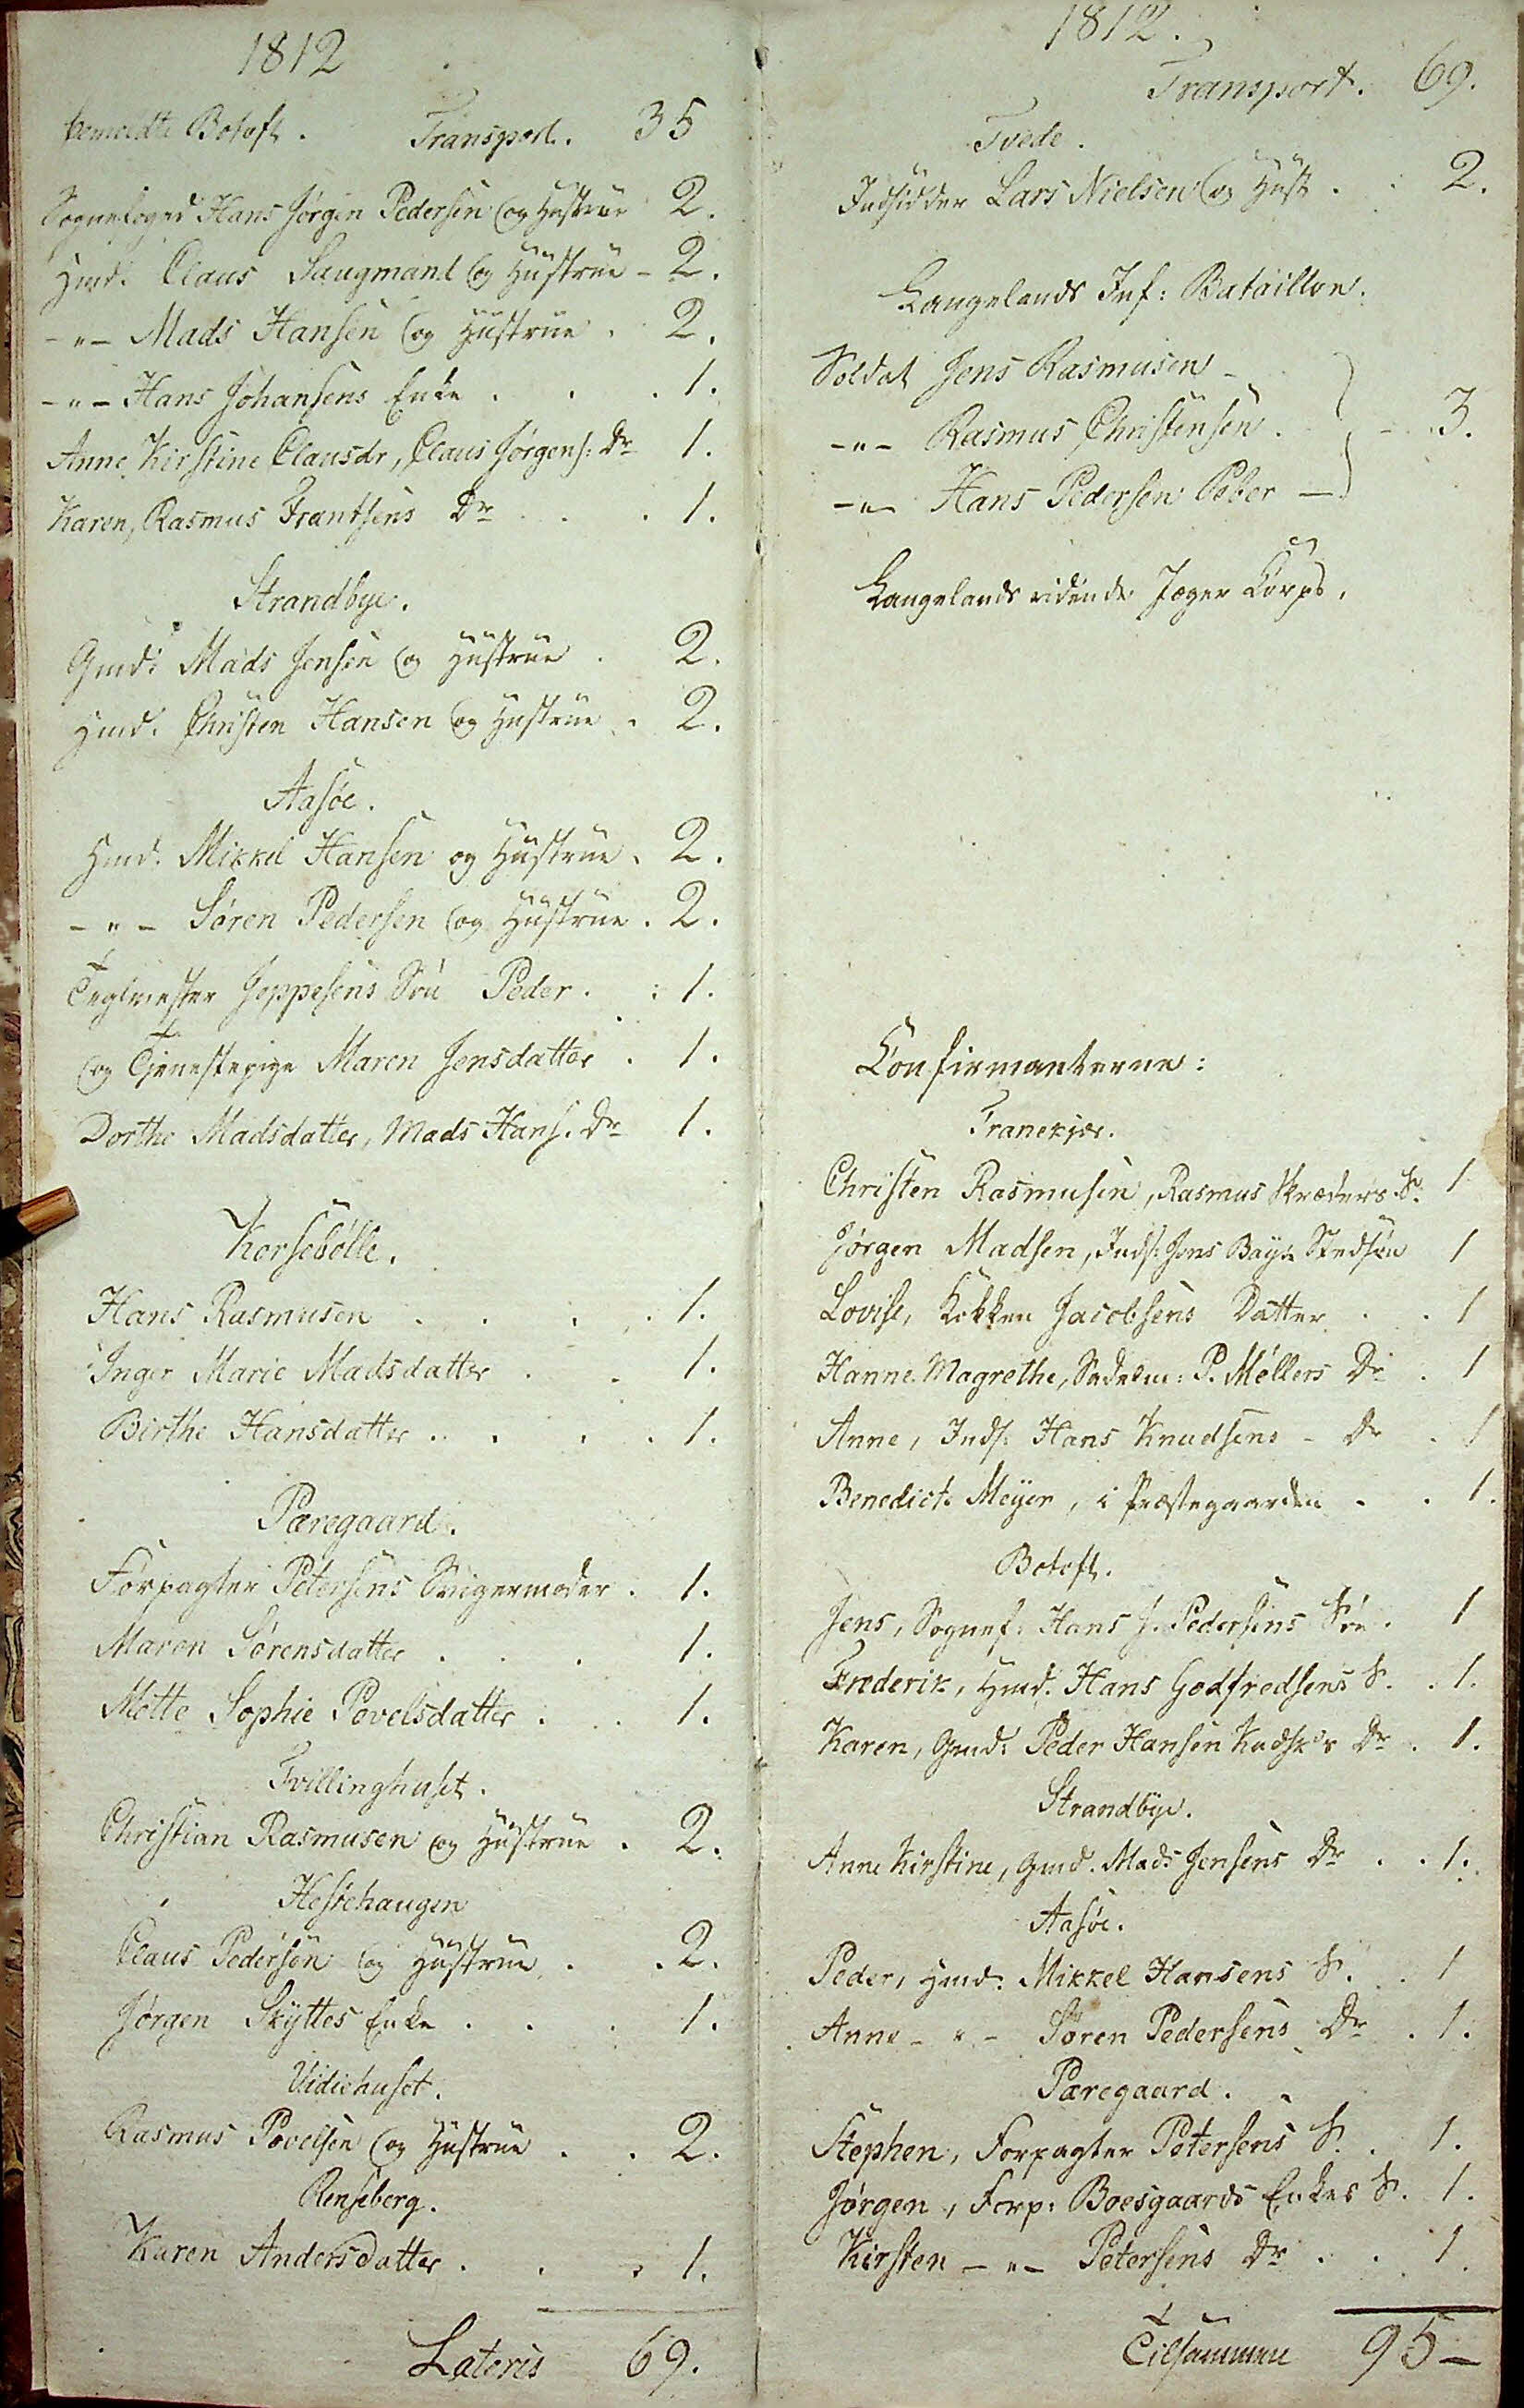1812
bemeldte Botoft. Transport. 35
Sognefoged Hans Jørgen Pedersen og Hustrue 2.
Hmd: Claus Saugmand og Hustrue - 2.
-"- Mads Hansen og Hustrue . 2.
-"- Hans Johansens Enke  . . . 1.
Anne Kirstine Clausdr, Claus Jørgens: Dr 1.
Karen, Rasmus Frantzens Dr . . . 1.
Strandbye.
Gmd. Mads Jensen og Hustrue . 2.
Hmd. Christen Hansen og Hustrue . 2.
Aasøe
Hmd. Mikkel Hansen og Hustrue . 2.
-"- Søren Pedersen og Hustrue . 2.
Teglmester Jeppesens Søn Peder . : 1.
og Tjenestepige Maren Jensdatter . 1.
Dorthe Madsdatter, Mads Hans. Dr 1.
Korsebølle.
Hans Rasmusen . . . . 1.
Inger Marie Madsdatter . . 1.
Birthe Hansdatter . . . . 1.
Pæregaard.
Forpagter Petersens Svigermoder . 1.
Maren Sørensdatter . . . 1.
Mette Sophie Povelsdatter . . . 1.
Tvillinghuset
Christian Rasmusen og Hustrue . 2.
Hestehaugen
Claus Pedersens og Hustrue . . 2.
Jørgen Skyttes Enke . . . 1.
Vidiehuset.
Rasmus Povelsen og Hustrue . . 2.
Renseberg.
Karen Andersdatter . . . 1.
Lateris 69.
1812
Transport. 69.
Tvede
Indsidder Lars Nielsens og Hust . . 2.
Langelands Inf: Bataillon.
Soldat Jens Rasmusen
-"- Rasmus Christensen
-"- Hans Pedersen ##ber
3.
Langelands ridende Jæger Corps.
Konfirmanterne:
Tranekjær
Christen Rasmusen, Rasmus Skræders S . 1
Jørgen Madsen, Jnds: Jens Bays Stedsøn 1
Lovise, Kokken Jacobsens Datter . . 1
Hanne Magrethe, Sadelm: P. Møllers Dr 1
Anne, Inds: Hans Knudsens - Dr . 1
Bendicte Meyer, i Præstegaarden . . 1
Botoft.
Jens, Sognef: Hans J. Pedersens Søn . 1
Frederik, Hmd. Hans Godfredsens S . . 1.
Karen, Gmd: Peder Hansen Kudsk's Dr . 1.
Stranbye.
Anne Kirstine, Gmd. Mads Jensens Dr . . 1.
Aasøe.
Peder, Hmd Mikkel Hansens S . . . 1
Anne -"- Jøren Pedersens Dr . 1.
Pæregaard.
Stephen, Forpagter Petersens S . . 1.
Jørgen, Forp: Boesgaards Enkes S .1.
Kirsten -"- Petersens Dr . . 1.
Tilsammen 95 -
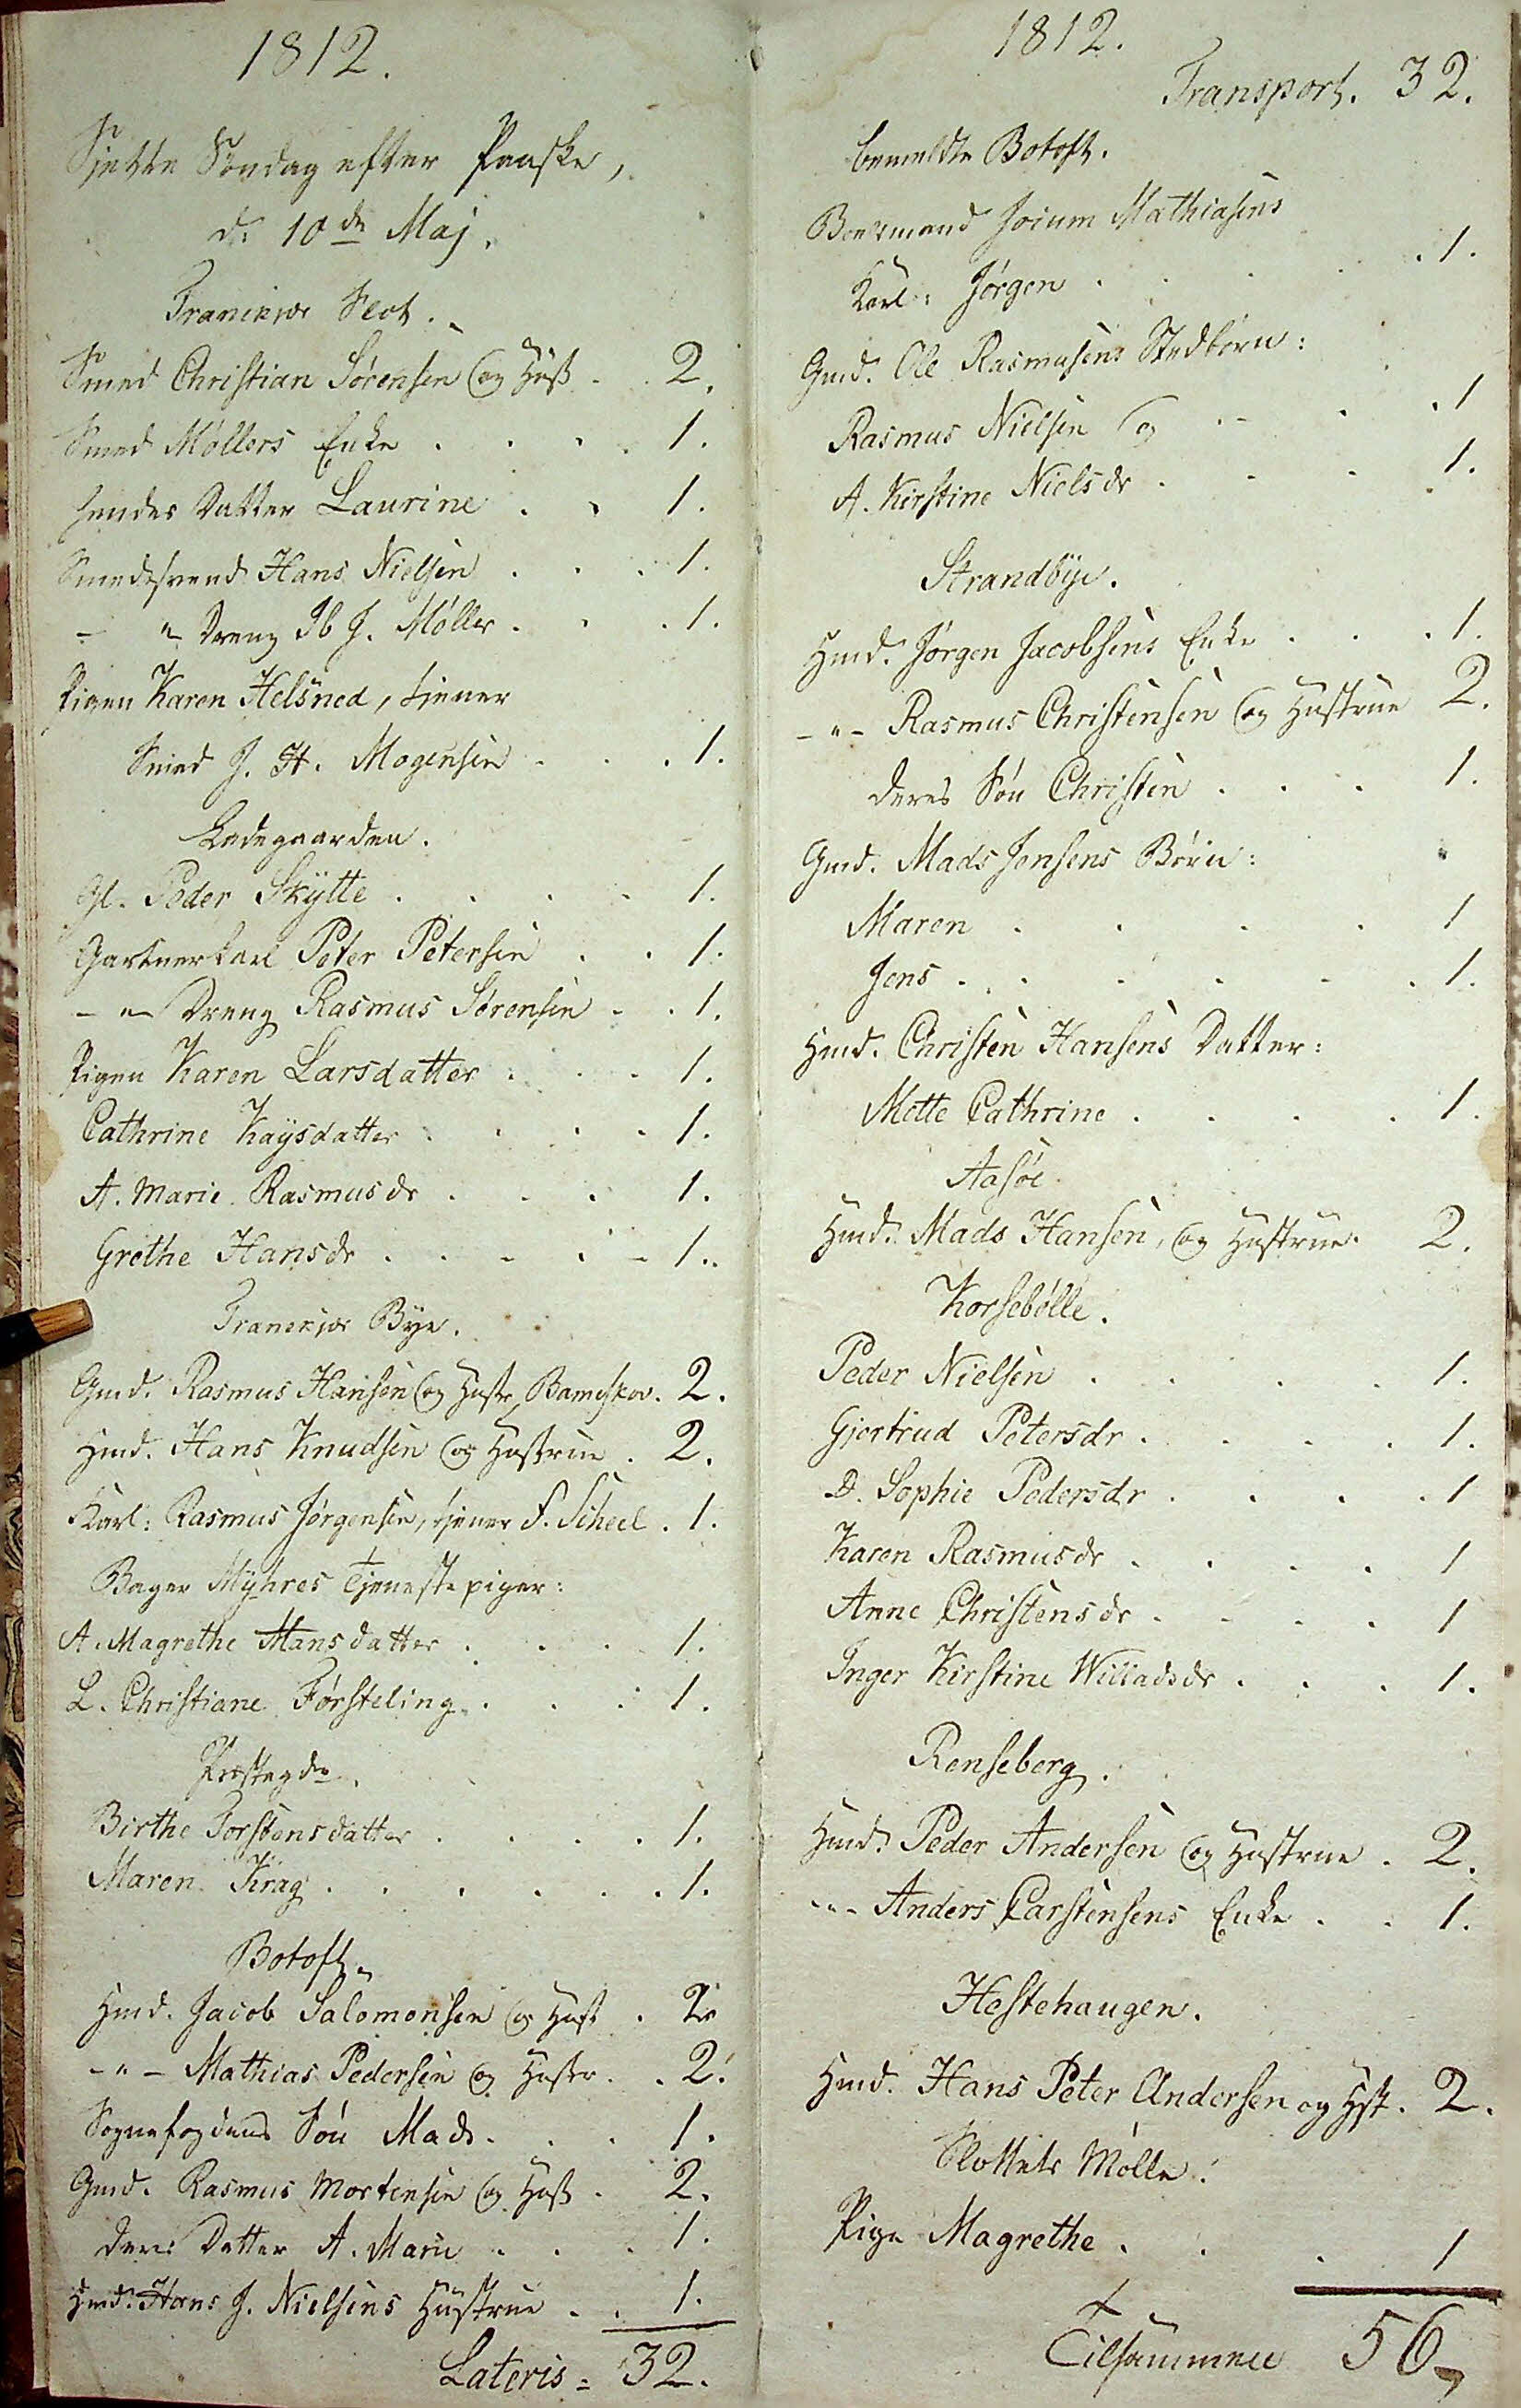1812.
Sjette Søndag efter Paaske,
d. 10de Maj
Tranekjær Slot.
Smed Christian Sørensen og Hust . . 2.
Smed Møllers Enke . . . . 1.
hendes Datter Laurine . . 1.
Smedsvend Hans Nielsen . . . 1.
Dreng Ib J. Møller . . . 1.
Pigen Karen Helsned, tjener
Smed J. H. Mogensen . . . 1
Ladegaarden.
Gl. Peder Skytte . . . . 1.
Gartnerkarl Peter Petersen . . 1
-"- Dreng Rasmus Sørensen . . 1.
Pigen Karen Larsdatter . . . 1.
Cathrine Kaysdatter . . . . 1.
A. Marie Rasmusdr . . . 1
Grethe Hansdr . . . . . 1.
Tranekjær Bye.
Gmd. Rasmus Hansen og Hustr Bameskov . 2.
Hmd. Hans Knudsen og Hustrue . 2.
Karl: Rasmus Jørgensen, tjener F. Schell . 1.
Bager Myhres Tjenestepiger:
A. Magrethe Hansdatter . . . 1.
L. Christiane Førsteling . . . 1.
Præstegdr
Birthe Torstensdatter . . . . 1.
Maren Krag . . . . . . 1.
Botoft.
Hmd Jacob Salomonsen og Hust . 2.
-"- Mathias Pedersen og Hustr . . 2.
Sognefogdens Søn Mads . . . 1.
Gmd. Rasmus Mortensen og Hust . 2.
deres Datter A. Marie . . . 1.
Hmd. Hans J. Nielsens Hustrue . . 1.
Lateris = 32.
1812.
Transport. 32.
bemeldte Botoft.
Boelsmand Jocum Mathiasens
Karl: Jørgen . . . . . 1.
Gmd: Ole Rasmusens Stedbørn:
Rasmus Nielsen og . . . . 1
A. Kirstine Nielsdr . . . 1.
Stranbye.
Hmd. Jørgen Jacobsens Enke . . . 1.
-"- Rasmus Christensen og Hustrue 2.
deres Søn Christen . . . 1.
Gmd. Mads Jensens Børn:
Maren . . . . 1
Jens . . . . . . 1.
Hmd: Christen Hansens Datter:
Mette Cathrine . . . . 1.
Aasøe
Hmd. Mads Hansen, og Hustrue . 2.
Korsebølle.
Peder Nielsen . . . . 1
Gjertrud Pedersdr . . . . 1.
D. Sophie Pedersdr . . . . 1
Karen Rasmusdr . . . . 1
Anne Christensdr . . . . 1
Inger Kirstine Willadsdr . . . 1.
Renseberg
Hmd. Peder Andersen og Hustrue . 2.
-"- Anders Carstensens Enke . . 1.
Hestehaugen.
Hmd. Hans Peter Andersen og Hstr . 2.
Slottets Mølle.
Pige Magrethe . . . 1
Tilsammen 56.